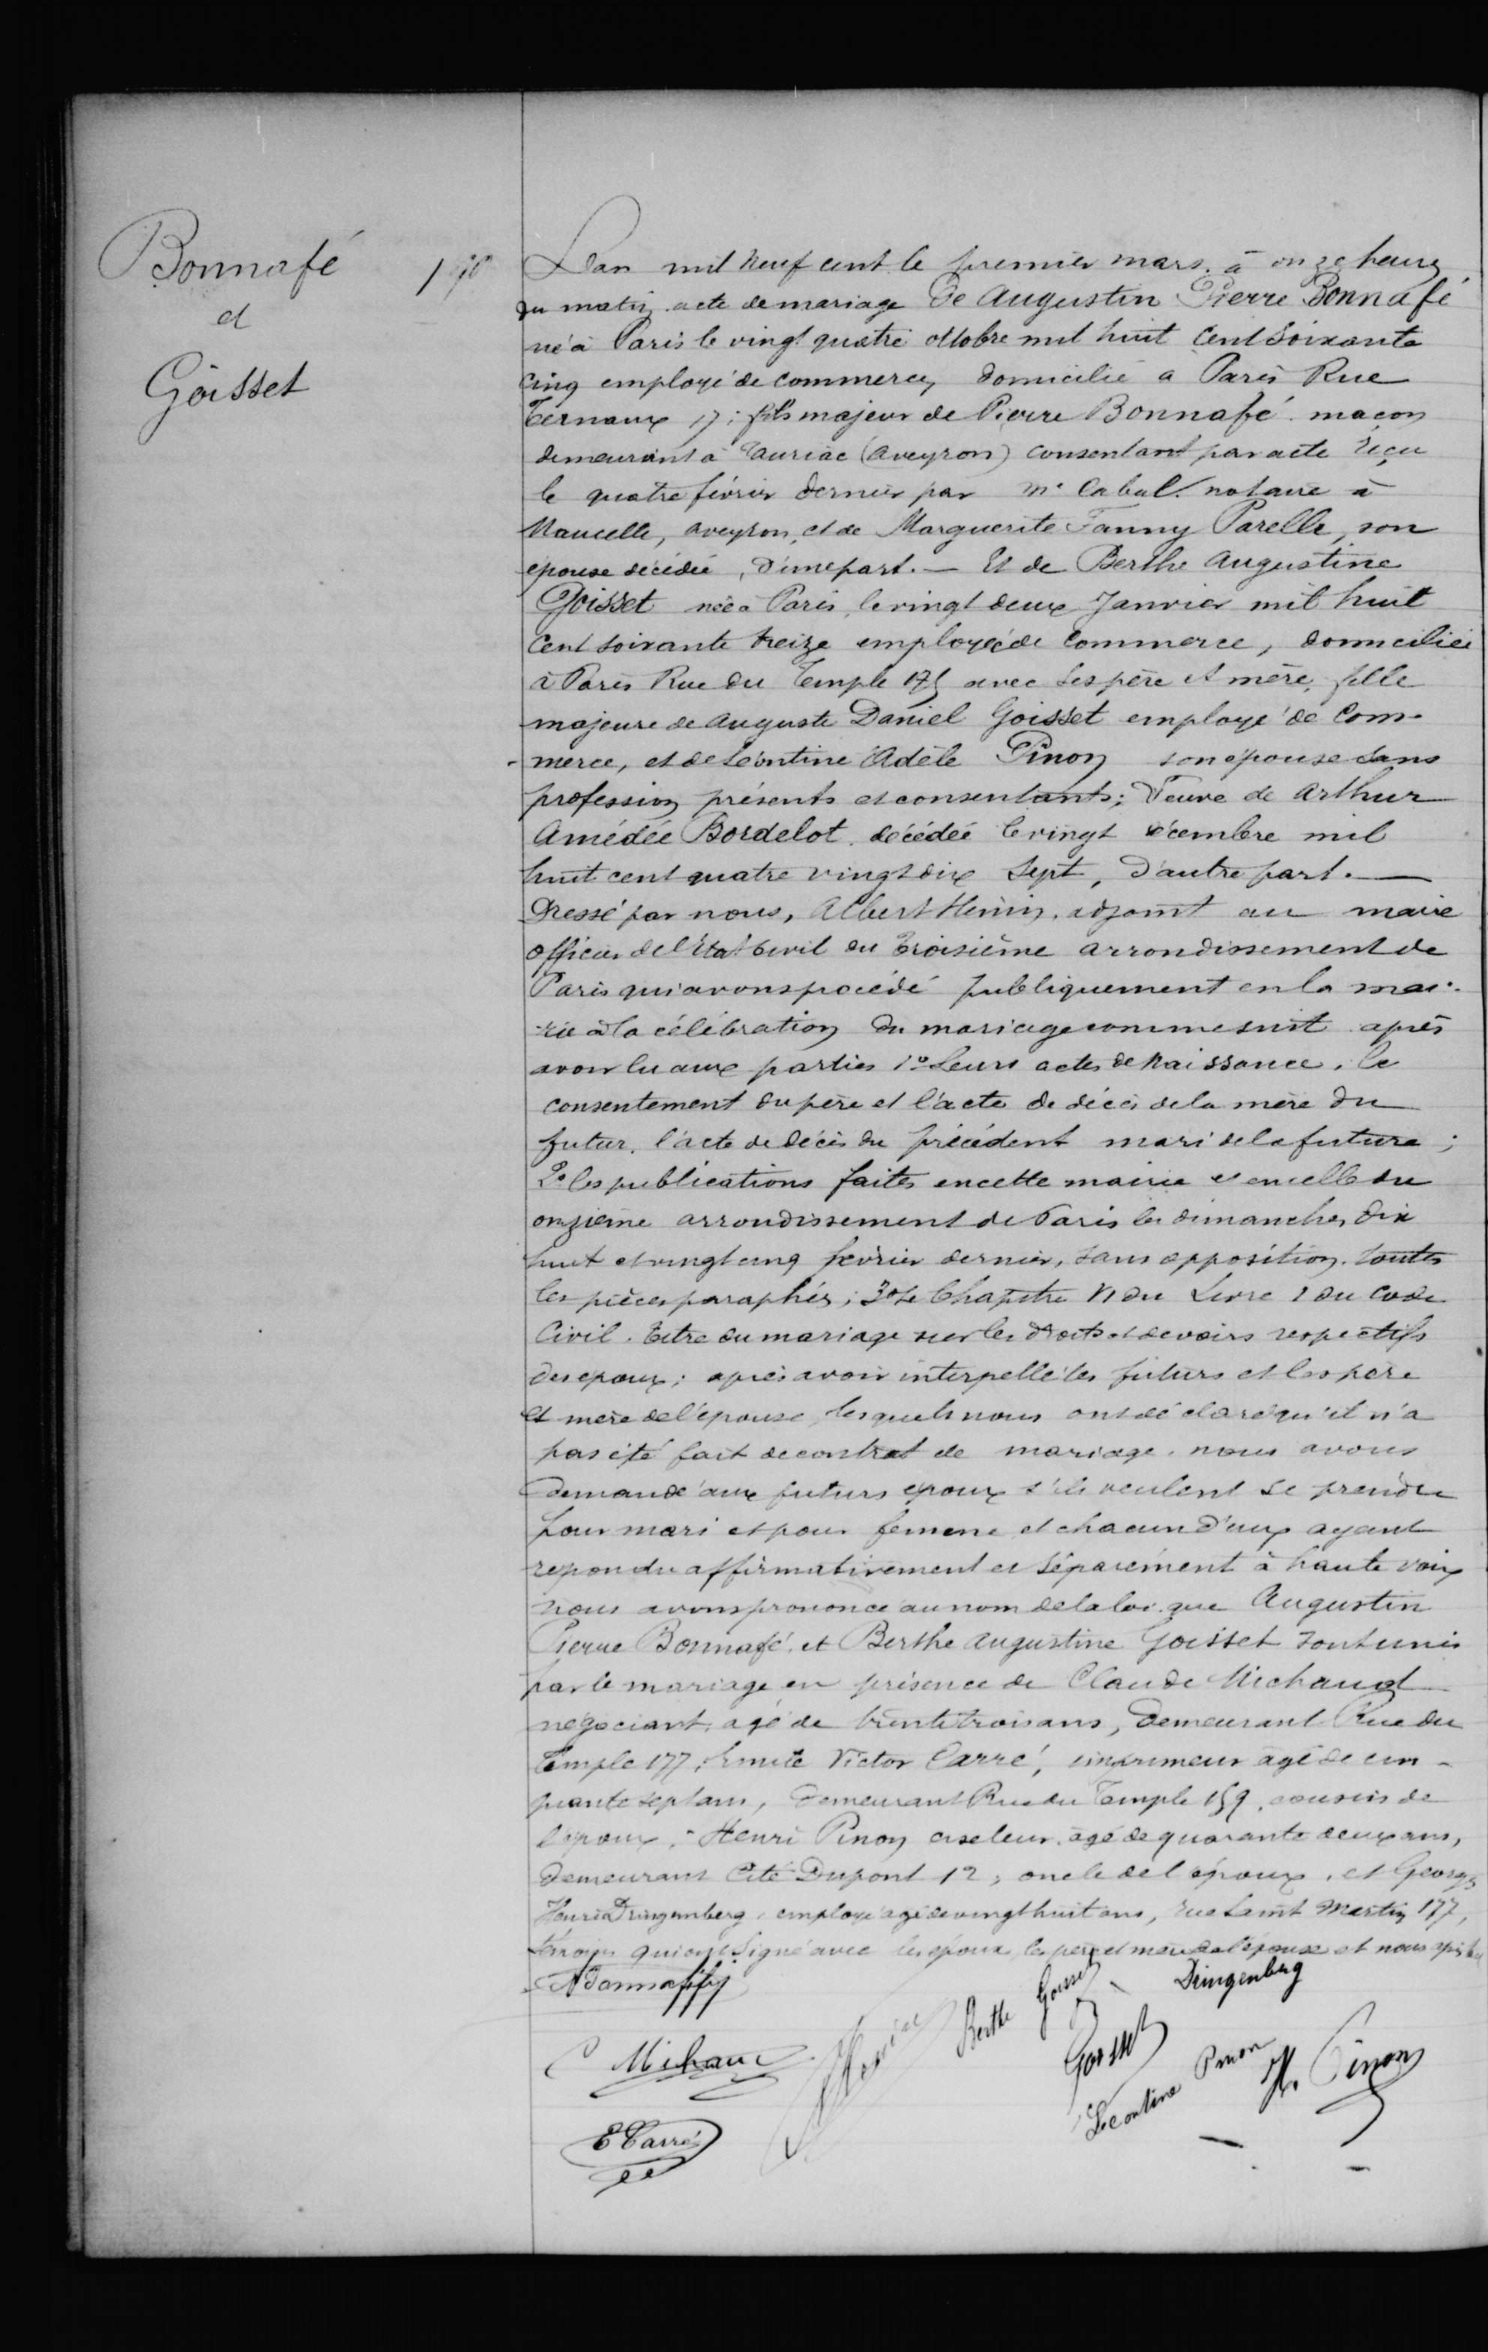Bonnafé 190
et
Goisset
L'an mil neuf cent le premier mars à onze heures
du matin acte de mariage de Augustin Pierre Bonnafé
né à Paris le vingt quatre octobre mil huit cent soixante
cinq employé de commerce domicilié à Paris Rue
Ternaux 17: fils majeur de Pierre Bonnafé maçon
demeurant à Auriac (Aveyron) consentant par acte reçu
le quatre février dernier par M° Cabul notaire à
Maucelle, aveyron, et de Marguerite Fanny Parelle, son
épouse décédée, d'une part. -Et de Berthe Augustine
Goisset née à Paris, le vingt deux Janvier mil huit
cent soixante treize employée de commerce, domiciliée
à Paris Rue du Temple 175 avec les père et mère, fille
majeure de Auguste Daniel Goisset employé de com-
merce, et de Léontine Adèle Pinon son épouse sans
profession présents et consentants; Veuve de arthur
Amédée Bordelot, décédée le vingt décembre mil
huit cent quatre vingt dix sept, d'autre part .-
Dressé par nous, Albert Hénin, adjoint au maire
officier de l'état civil du troisième arrondissement de
Paris qui avons procédé publiquement en la mai-
rie à la célébration du mariage comme suit après
avoir lu aux parties 1° leurs actes de naissance, le
consentement du père et l'acte de décès de la mère du
futur, l'acte de décès du précédent mari de la future;
2° les publications faites en cette mairie et en celle du
onzième arrondissement de Paris les dimanches dix
huit et vingt cinq février dernier, sans opposition, toutes
les pièces paraphés; 3° le Chapitre VI du Livre I du code
Civil titre du mariage sur les droits et devoirs respectifs
des epoux; après avoir interpellé les futurs et les père
et mère de l'époux, lesquels nous ont déclaré qu'il n'a
pas été fait de contrat de mariage, nous avons
demandé aux futurs époux s'ils veulent se prendre
pour mari et pour femme et chacun d'eux ayant
repondu affirmativement et séparément à haute voix
nous avons prononcé au nom de la loi que Augustin
Pierre Bonnafé et Berthe Augustine Goisset sont unis
par le mariage en présence de Claude Michaud
négociant agé de trente trois ans, demeurant Rue du
Temple 177, Emile Victor Carré, imprimeur âgé de cin-
quante sept ans, demeurant Rue du Temple 159, cousin de
l'époux-Henri Pinon ciseleur, âgé de quarante deux ans,
demeurant Cité Dupont 12; oncle de l'époux et Georges
Henri Dringemberg, employé agé de vingt huit ans, rue Saint Martin 177,
témoins qui ont signé avec les époux les père et mère de l'épouse et nous après
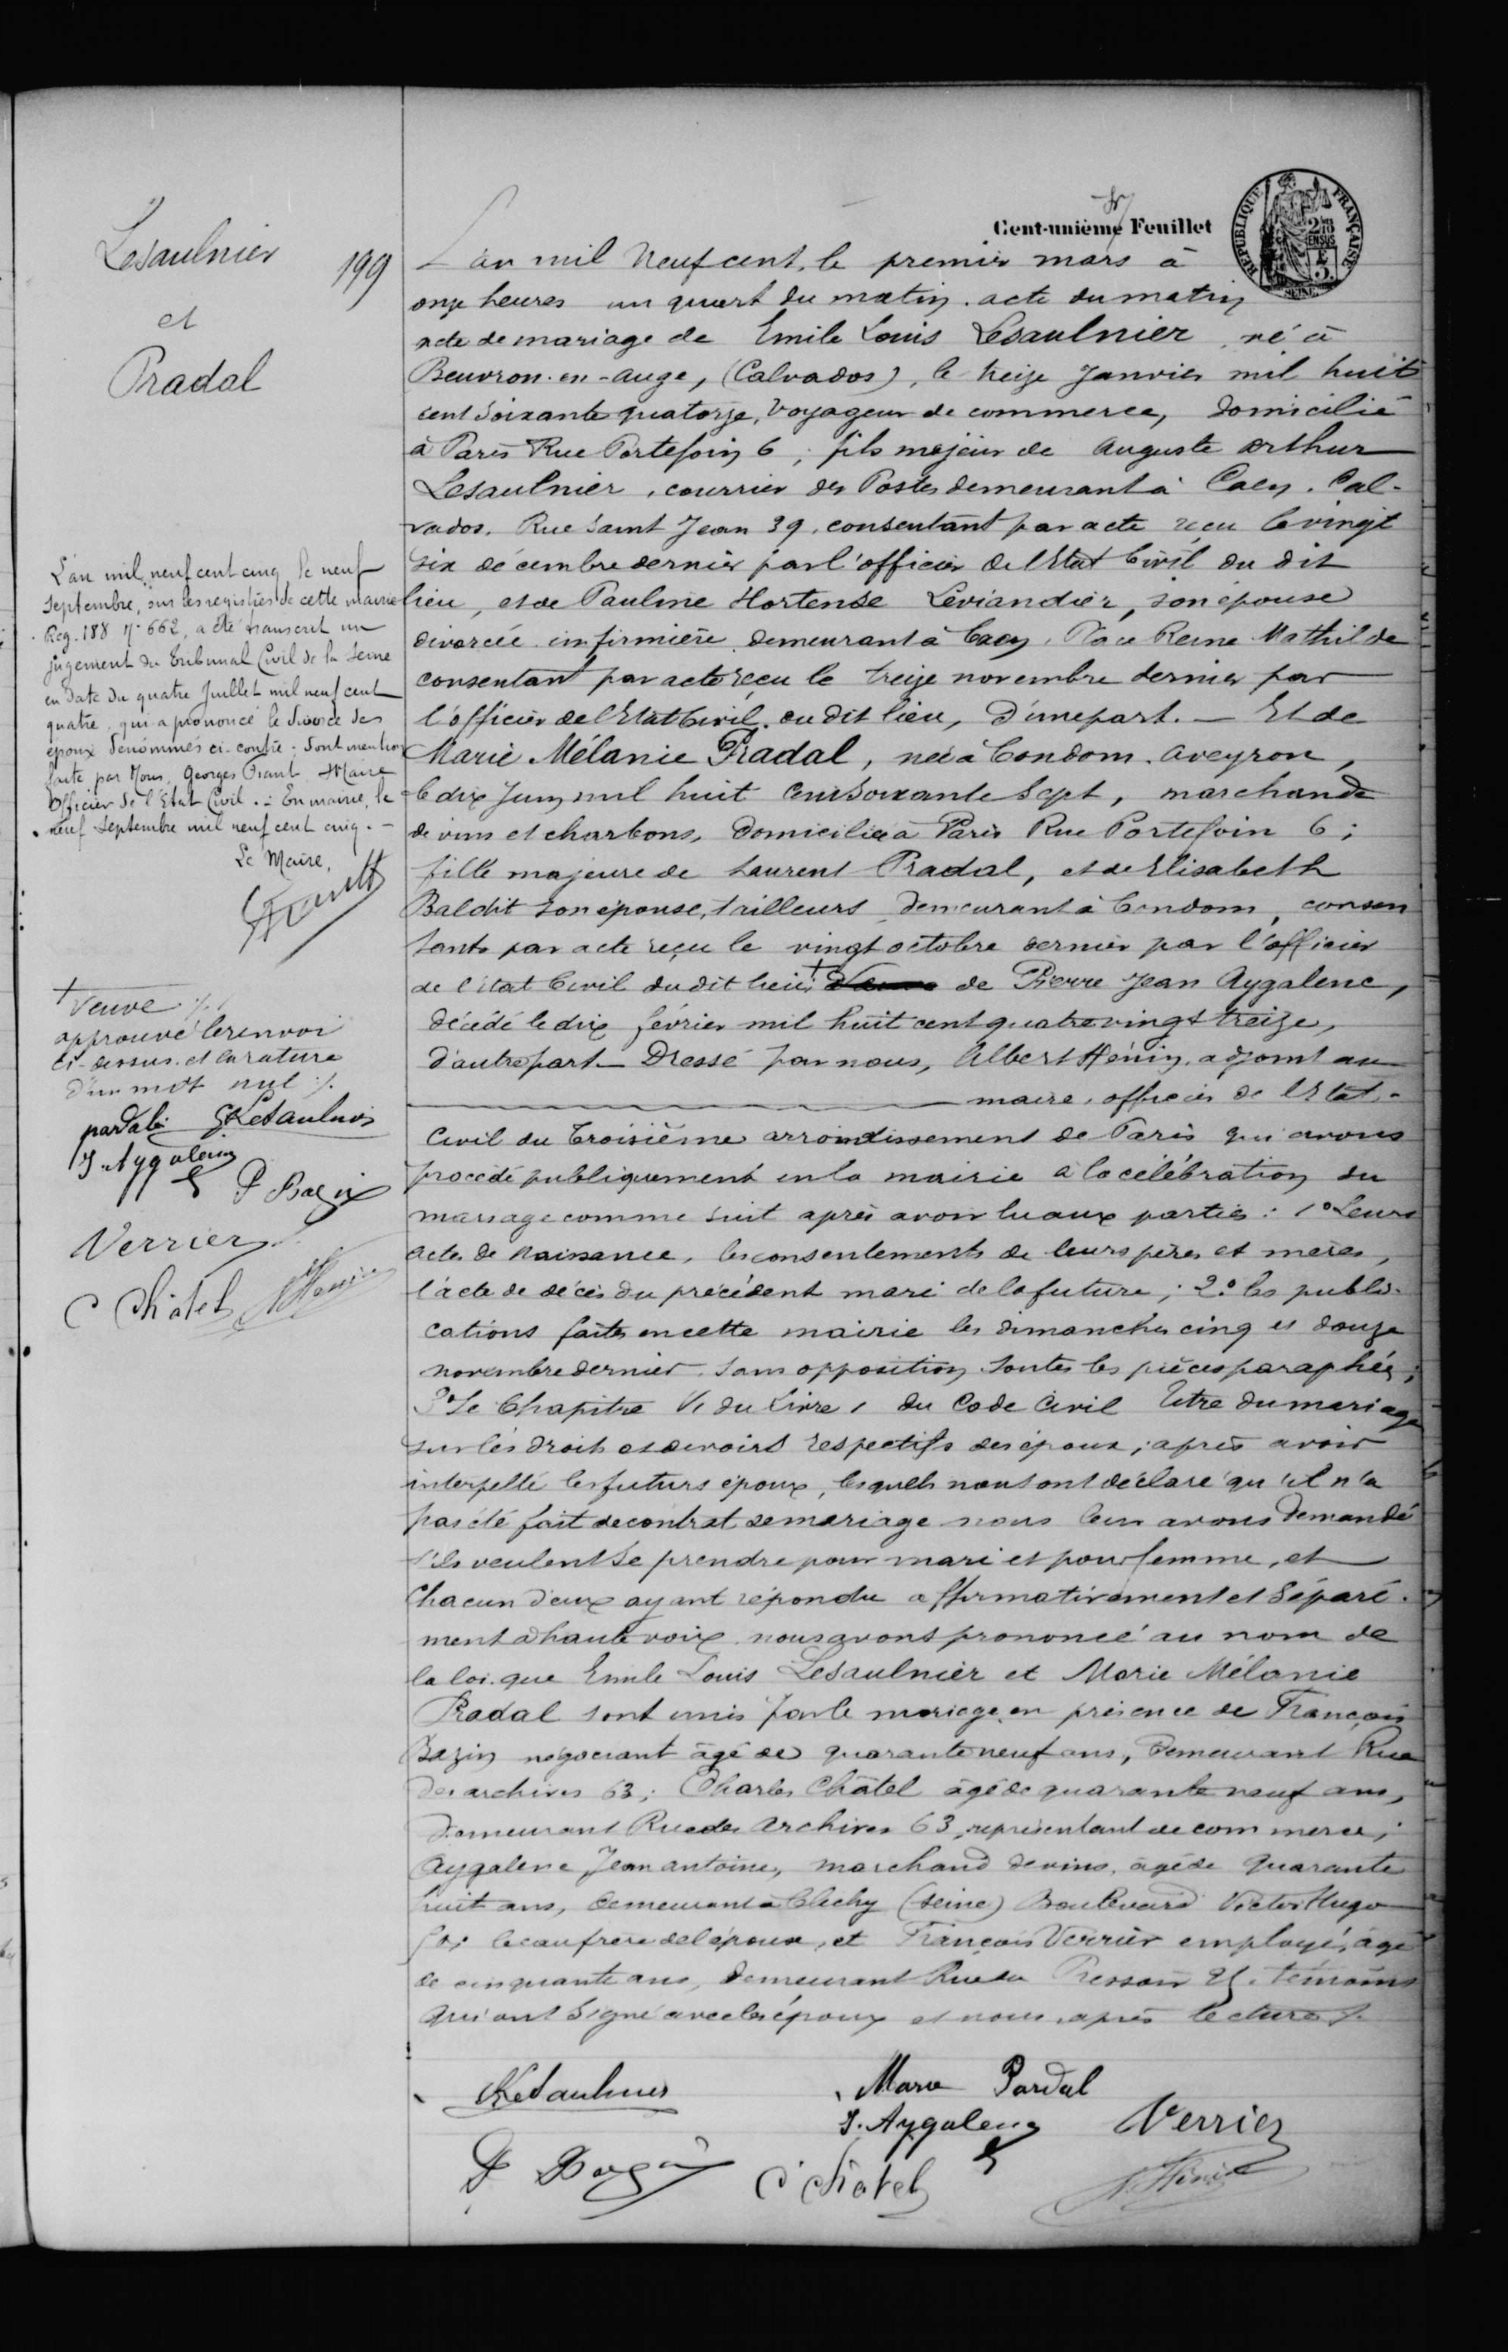Lesaulnier 199
et
Pradal
L'an mil neuf cent, le premier mars à
onze heures un quart du matin acte du matin
acte de mariage de Emile Louis Lesaulnier, né à
Beuvron-en-auge, (Calvados), le treize Janvier mil huit
cent soixante quatorze, voyageur de commerce, domicilié
à Paris Rue Portefois, 6; fils majeur de auguste arthur
Lesaulnier, courrier des Postes demeurant à Caen, Cal
vados. Rue Saint Jean 39, consentant par acte reçu le vingt
six décembre dernier par l'officier de l'Etat Civil du dit
lieu, et de Pauline Hortense Leviandier, son épouse
divorcée infirmière demeurant à Caen, Place Reine Mathilde
consentant par acte reçu le Treize novembre dernier par
l'officier de l'Etat Civil du dit lieu, d'une part. -Et de
Marie Mélanie Radal, née à Condom aveyron
le dix Juin mil huit cent soixante sept, marchande
de vin et charbons, domiciliée à Paris Rue Portefoin 6;
fille majeure de Laurent Pradal, et de Elisabeth
Baldit son épouse, tailleurs, demeurant à Condom, consen
tant par acte reçu le vingt octobre dernier par l'officier
de l'état Civil du dit lieu de Pierre Jean Aygalence,
décédé le dix février mil huit cent quatre vingt treize,
d'autre part -Dressé par nous, Albert Hénin, adjoint au
-maire, officier de l'Etat
Civil du Troisième arrondissement de Paris qui avons
procédé publiquement en la mairie à la célébration du
mariage comme suit après avoir lu aux parties: 1° Leurs
actes de naissance, les consentements de leurs pères et mères,
l'acte de décès du précédent mari de la future; 2° les publi
cations faites en cette mairie les dimanches cinq et douze
novembre dernier sans opposition toute les pièces paraphées;
3° Le Chapitre VI du Livre I du Code Civil Titre du mariage
sur les droits et devoirs respectifs des époux; après avoir
interpellé les futurs époux, lesquels nous ont déclaré qu'il n'a
pas été fait de contrat de mariage nous leur avons demandé
s'ils veulent se prendre pour mari et pour femme, et
chacun d'eux ayant répondu affirmativement et séparé
ment à haute voix nous avons prononcé au nom de
la loi que Emile Louis Lesaulnier et Marie Mélanie
Radal sont unis par le mariage en présence de François
Bezin négociant âgé de quarante neuf ans, demeurant Rue
des archives 63; Charles Châtel âgé de quarante neuf ans,
demeurant Rue des archives 63, représentant de commerce;
Aygalence Jean Antoine, marchand de vins âgé de quarante
huit ans, demeurant à Clichy (Seine) Boulevard Victor Hugo
50; beau frère de l'époux, et François Verrier employé, âgé
de cinquante ans, demeurant Rue du Pressoir 25. témoins
qui ont signé avec les époux et nous après lecture ./.